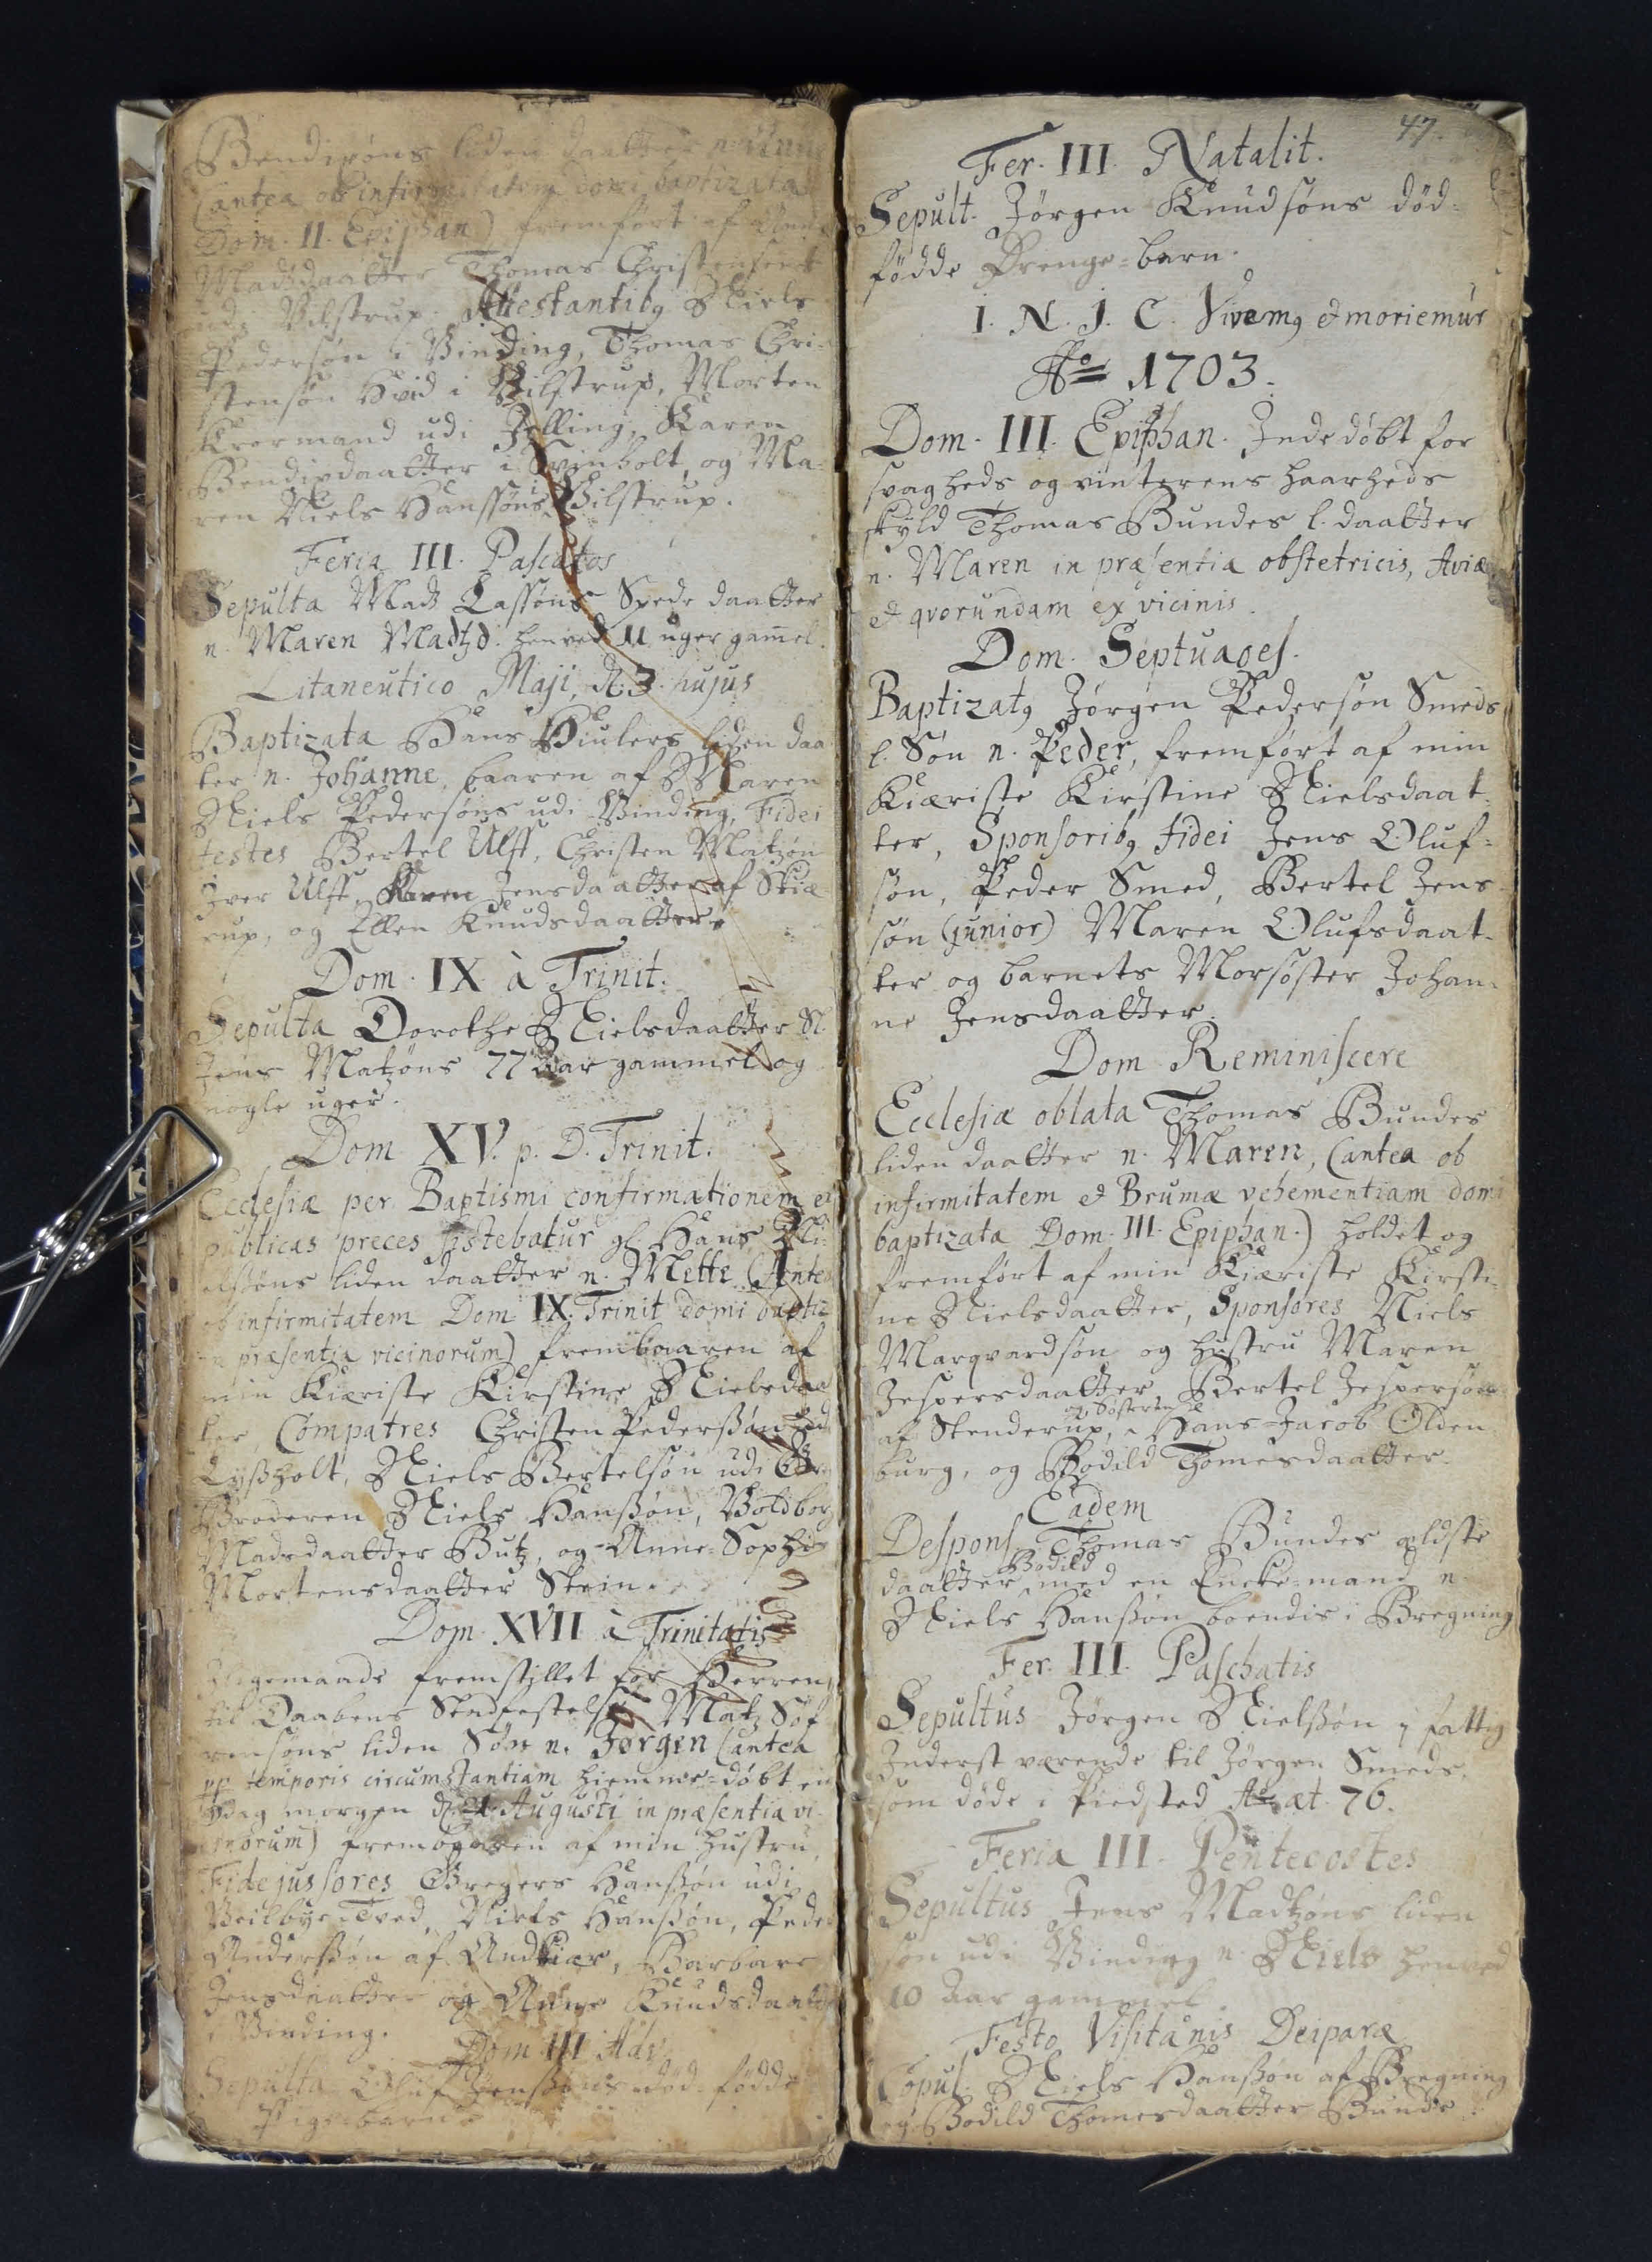Bendixsøns liden datter n. Anne
(antea ob infirmitatem domi baptizata
Dom. II. Epiphan) fremført af Anne
Madzdaatter Thomas Christensøns
udi Vilstrup. Attestantibq Niels
Pedersøn i Vinding, Thomas Chri¬
stensøn Hvid i Vilstrup, Morten
Krormand udi Jelling, Karen
Bendixdaatter i Svinholt, og Ma¬
i
ren Niels Hanssøns Vilstrup.
Feria III. Paschatos
Sepulta Madz Lassøns Spede daatter
n. Maren Madtzd. henved 11 uger gammel
Litaneutico Maji d. 3. hujus
Baptizata Hans Hiulers liden daat
ter n. Johanne, baaren af Maren
Niels Pedersøns udi Vinding. Fidei
testes Bertel Ulff, Christen Matzøn
Iver Ulff Karen Jensdaatter af Skiæ¬
rup, og Ellen Knudsdaatter.
Dom. IX. a Trinit.
Sepulta Dorothe Nielsdaatter Sl.
Jens Matzøns 77 Aar gammel og
nogle uger.
Dom. XV. p. D. Trinit.
Ecclesiæ per Baptismi confirmationem et
publicas preces sistebatur gl. Hans Ni¬
elsøns liden daatter n. Mette, (antea
ob infirmitatem Dom. IX Trinit. domi baptiz 
## præsentia vicinorum) frembaaren af
min Kiæriste Kirstine Nielsdaa 
ter, Compatres Christen Pederssøn ud 
Lyssholt, Niels Bertelsøn udi Gr##
Broderen Niels Hanssøn, Voldborg
Madsdaatter Butz, og Anne=Sophie
Mortensdaatter Stein.
Dom. XVII a Trinitatis
I ligemaade fremstillet for Herren
til Daabens Stedfestelse Matz Søf
rensøns liden Søn n. Jørgen (antea
pp temporis circumstantiam hiemme=døbt en
morgen d. 21 Augusti in præsentia vi
cinorum) frembaaren af min hustru
Fide jussores Gregers Hanssøn udi
Veilbye Tved, Niels Hanssøn, Peder
Anderssøn af Andkiær, Barbare
Jensdaatter og Anne Knudsdaatter
i Vinding.
Dom. III Adv
Sepulta Oluf Jenssøns død=fødde
Pige=barn.
47
Fer. III Natalit.
Sepult. Jørgen Knudsøns død¬
fødde Drenge=barn
I. N. J. C. Vivemq et moriemur
Ao 1703
Dom. III. Epiphan. Indedøbt for
svagheds og vinterens haarheds
skyld Thomas Bundes l. daatter
n. Maren, in præsentia obstetricis, Aviæ
et qvorundam ex vicinis
Dom. Septuages.
Baptizatq Jørgen Pedersøn Smeds
l. Søn n. Peder, fremført af min
Kiæriste Kirstine Nielsdaat¬
ter, Sponsoribq fidei Jens Oluf¬
søn, Peder Smed, Bertel Jens¬
søn. (junior) Maren Olufsdaat¬
ter og barnets Morsøster Johan¬
ne Jensdaatter
Dom Reminiscere
Ecclesiæ oblata Thomas Bundes
liden daatter n. Maren, (antea ob
infirmitatem et Brumæ vehementiam domi
baptizata Dom. III Epiphan.) holdt og 
fremført af min Kiæriste Kirsti¬
ne Nielsdaatter, Sponsores Niels
Marqvardsøn og hustru Maren
Jespersdaatter, Bertel Jespersøn
og## Søsteren## 
af Stenderup, Hans=Jacob Olden
burg, og Bodild Thomasdaatter.
Eadem
Despons Thomas Bundes ældste 
Bodild
daatter med en Encke=mand n.
Niels Hanssøn boendis i Breining
Fer. III. Paschatis.
Sepultus Jørgen Nielssøn 1 fattig
Inderst værende til Jørgen Smeds,
som døde i Piedsted Ao æt. 76.
Feria III. Pentecostes
Sepultus Jens Madtzøns liden 
søn udi Vinding n. Niels henved 
10 Aar gammel.
Festo Visitanis Deiparæ.
Copul. Niels Hanssøn af Breining
og Bodild Thomasdaatter Bundis
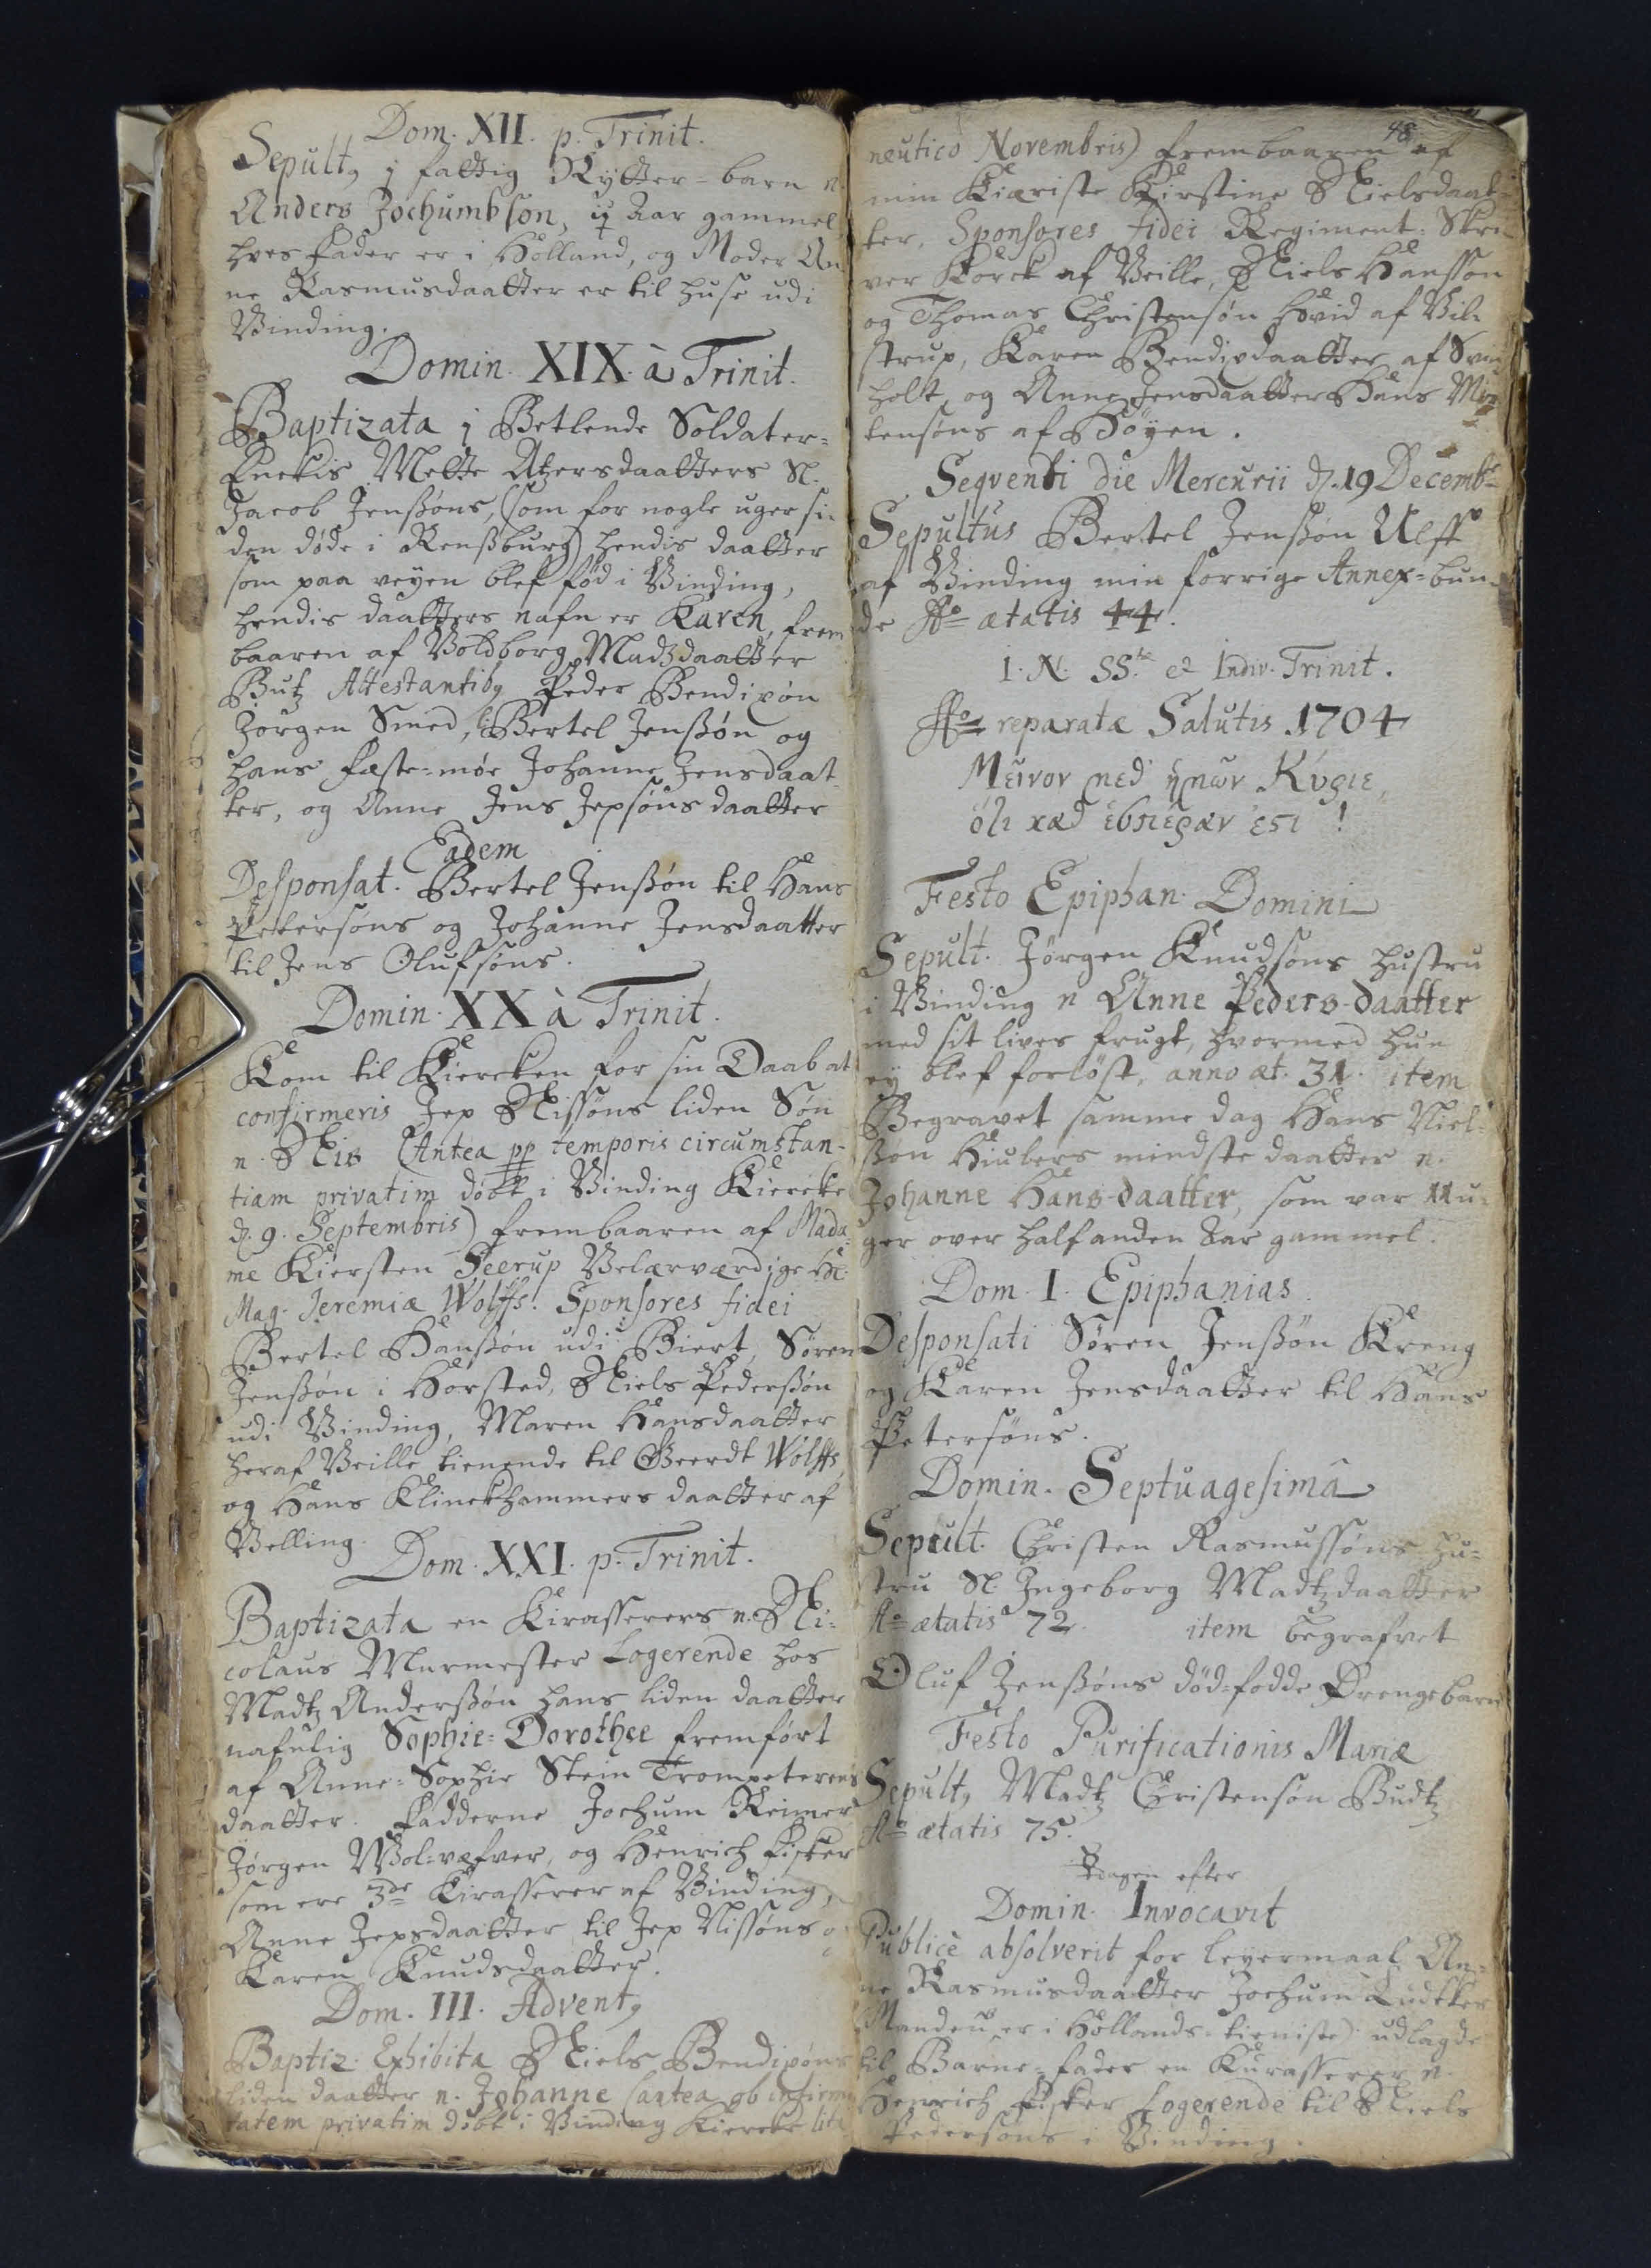Dom. XII. p. Trinit.
Sepultq 1 fattig Rytter-barn n.
Anders Jochumbsøn, 2 Aar gammel
hves Fader er i Holland, og Moder An¬
ne Rasmusdaatter er til huse udi
Vinding
Domin. XIX. a Trinit.
Baptizata 1 Betlende Soldater=
Enckis Mette Atzersdaatter Sl.
Jacob Jenssøns, (som for nogle uger si¬
den døde i Renssburg) hendis daatter 
som paa weyen blef fød i Vinding
hendis daatters nafn er Karen, frem
baaren af Voldborg Madtzdaatter
Butz Attestantibq Peder Bendixøn
Jørgen Smed, l. Bertel Jenssøn og
hans fæste=møe Johanne Jensdaat
ter, og Anna Jens Jepsøns daatter.
Eadem
Desponsat Bertel Jenssøn til Hans
Petersøns og Johanne Jensdaatter
til Jens Olufsøns
Domin. XX a Trinit.
Kom til Kircken for sin Daab at
confirmeris Jep Nissøns liden Søn 
n. Niss. (Antea pp temporis circumstan¬
tiam privatim døbt i Vinding Kircke
d. 9 Septembris) frembaaren af Mada¬
me Kiersten Seerup Velærværdige Hl.
Mag. Jeremiæ Wolffs. Sponsores fidei 
Bertel Hanssøn udi Biert, Søren
Jenssøn i Horsted, Niels Pederssøn
udi Vinding, Maren Hansdaatter
her af Veille tienende til Geerdt Wolffs
og Hans Klinckhammers daatter af 
Velling
Dom. XXI. p. Trinit.
Baptizata en Kirasserers n. Ni¬
colaus Murmester Logerende hos
Madtz Anderssøn hans liden daatter
nafnlig Sophie=Dorothee fremført
af Anne=Sophie Stein Trompeterens
daatter. Fadderne Jochum Reimer
Jørgen Wol=væfver, og Henrich Fisker
som ere 3de Kirasserer af Vinding
Anne Jepsdaatter til Jep Nissøns og
Karen Knudsdaatter
Dom. III. Adventq
Baptiz. Exhibita Niels Bendixøns
liden daatter n. Johanne, (antea ob in firmi
tatem privatim døbt i Vinding Kircke
48
neutica Novembris) frembaaren af
min Kiæriste Kirstine Nielsdaat¬
ter. Sponsores fidei Regiment=Skri¬
ver Korck af Veille, Niels Hanssøn
og Thomas Christensøn Hvid af Vil¬
trup, Karen Bendixdaatter af Svin¬
holt og Anne Jensdaatter Hans Mor
tensøns af Høyen
Seqvendi die Mercurii d. 19 Decembr
Sepultus Bertel Jenssøn Ulff
af Vinding min forrige Annex=bun¬
de Ao ætatis 44
I. N. SSta et Indiv Trinat.
Ao reparata Salutis 1704
Meivov ned## ## Kvqie
oli xad eboegav## esi
Festo Epiphan. Domini
Sepult. Jørgen Knudsøns hustru 
i Vinding n. Anne Pedersdaatter
med sit lives frugt, hvormed hun
ey blef forløst, anno æt. 31. item
Begravet samme dag Hans Niel¬
ssøn Hiulers mindste daatter n.
Johanne Hans-daatter, som var 11 u¬
ger over halfanden Aar gammel
Dom. I. Epiphanias
Desponsati Søren Jenssøn Kreng
og Karen Jensdaatter til Hans
Petersøns
Domin Septuagesima
Sepult. Christen Rasmussøns hu¬
stru Sl. Ingeborg Madtzdaatter 
Ao ætatis 72.
item begrafvet
Oluf Jenssøns død=fødde Drengebarn.
Festo Purificationis Mariæ
Sepultq Madtz Christensøn Budtz
Ao ætatis 75
☿ dagen efter
Domin. Invocavit
Publice absolverit for leyermaal An¬
ne Rasmusdaatter Jochum Ludtkes
NB
(Manden er i Hollands tieneste) udlagde
til Barne=fader en Kurasserer n.
Henrich Fisker, Logerende til Niels 
Pedersøns i Vinding